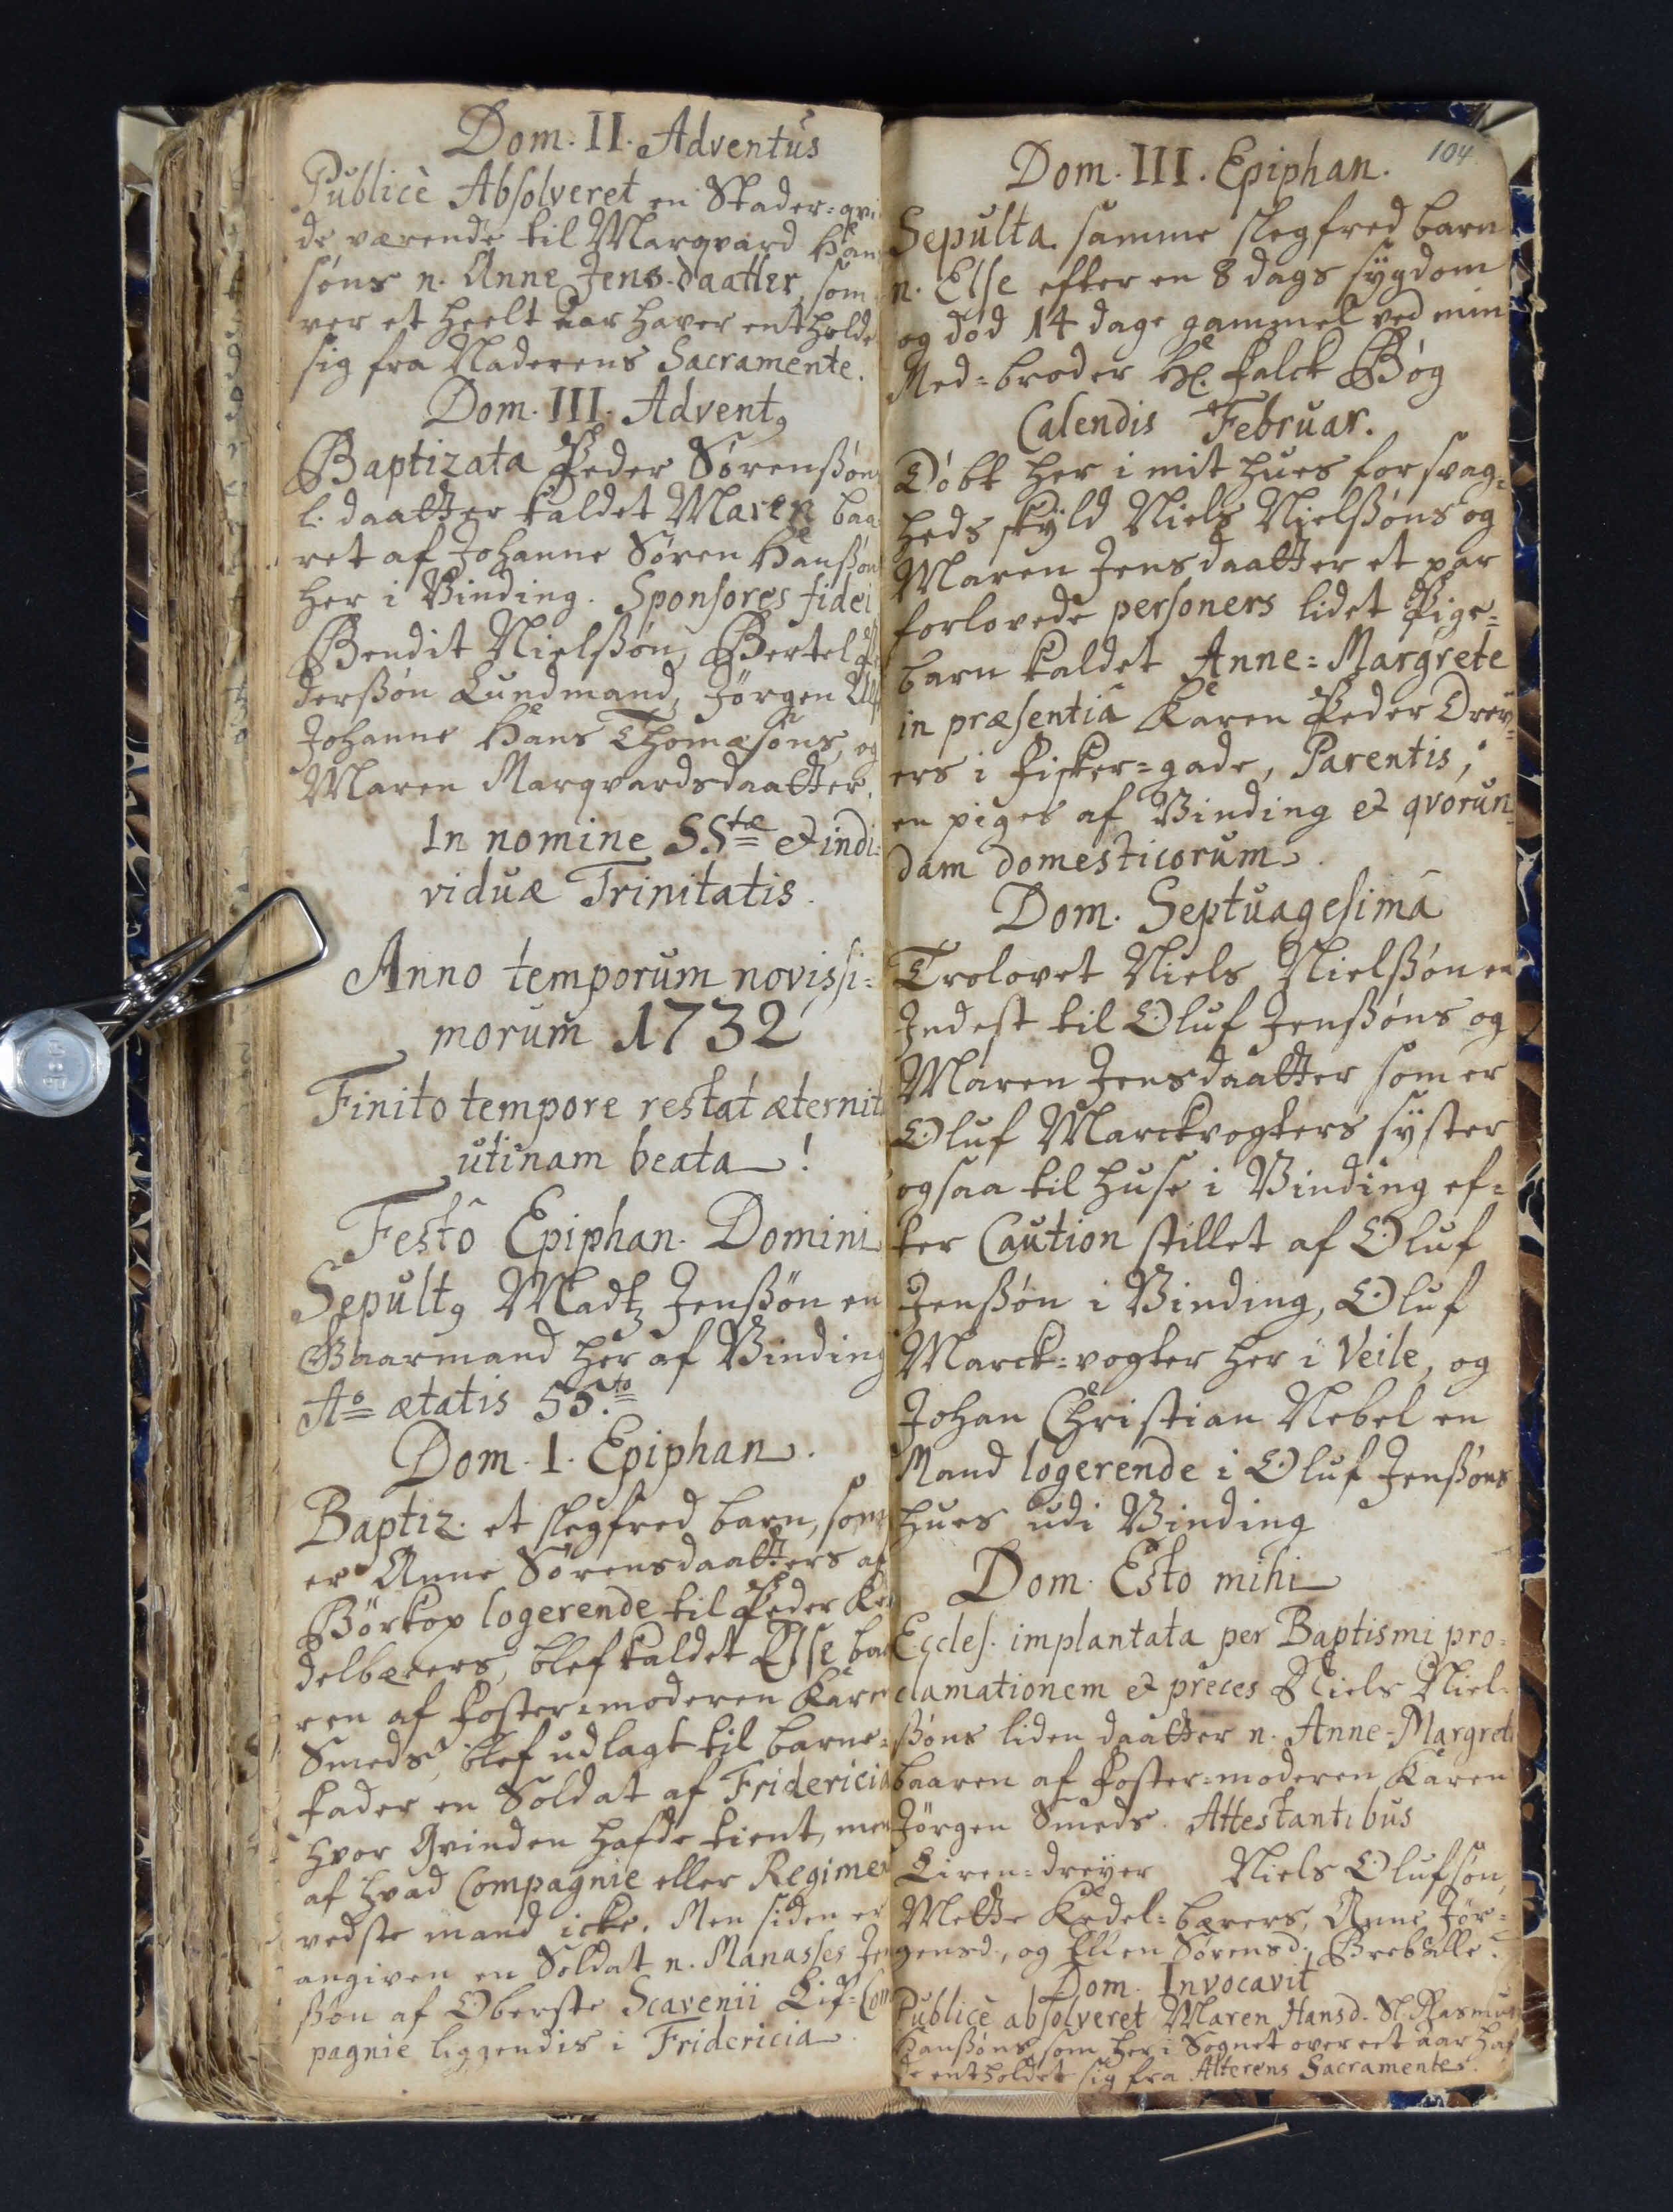Dom. II. Adventus
Publice Absolveret en Stader=qvi  
de værende til Marqvard Han
søns n. Anne Jens-daatter, som
ver et heelt Aar haver entholde  
sig fra Nadverens Sacramente.
Dom. III. Adventq
Baptizata Peder Sørenssøns
l. daatter kaldet Maren, baa¬
ret af Johanne Søren Hanssøn  
her i Vinding. Sponsores fidei
Bendit Nielssøn. Bertel Pe
derssøn Lundmand, Jørgen Ulf  
Johanne Hans Thomæsøns, og
Maren Marqvardsdaatter.
In nomine SStæ et indi¬
viduæ Trinitatis.
Anno temporum novissi¬
morum 1732
Finito tempore restat æternit
utinam beata!
Festo Epiphan. Domini
Sepultq Madtz Jenssøn en
Gaardmand her af Vinding
Ao ætatis 55to.
Dom. I. Epiphan.
Baptiz. et slegfred barn, som
er Anne Sørensdaatters af
Børcop logerende til Peder Ke  
delbærers, blef kaldet Else, bar  
ren af Foster=moderen Kare  
Smeds, blef udlagt til barne
fader en Soldat af Fridericia  
hvor Qvinden hafde tient, men  
af hvad Compagnie eller Regime  
vedste mand icke. Men siden er
angiven en Soldat n. Manasses Je  
ssøn af Oberste Scavenii Lif=Com  
pagnie liggendis i Fridericia
104
Dom. III. Epiphan.
Sepulta samme slegfred barn
n. Else, efter en 8 dages sygdom
og død 14 dage gammel ved min 
Med=broder Hl Falck Bøg
Calendis Februar.
Døbt her i mit hues for svag¬
heds skyld Niels Nielssøns og
Maren Jensdaatter et par
forlovede personers lidet Pige¬
barn kaldet Anne = Margrete
in præsentia Karen Peder Drey¬
ers i Fisker=gade, Parentis
en piges af Vinding et qvorun¬
dam domesticorum.
Dom. Septuagesima
Trolovet Niels Nielssøn en
Indest til Oluf Jenssøns og
Maren Jensdaatter som er
Oluf Marckvogters syster
ogsaa til huse i Vinding ef¬
ter Caution stillet af Oluf
Jenssøn i Vinding, Oluf
Marck=vogter her i Veile, og
Johan Christian Nebel en
Mads logerende i Oluf Jenssøns
hues udi Vinding
Dom. Esto mihi
Eccles. implantata per Baptismi pro¬
clamationem et preces Niels Niel¬
ssøns liden daatter n. Anne-Margret  
baarent af Foster=moderen Karen
Jørgen Smeds. Attestantibus
Liren=dreyer Niels Olufsøn,
Mette Kedel=bærers, Anne Jør¬
gensd., og Ellen Sørensd. Breballe.
Dom. Invocavit
Publice absolveret Maren Hansd. Sl. Rasmus
Hanssøns som her i Sognet over eet Aar haf
de entholdet sig fra Alterens Sacramente.
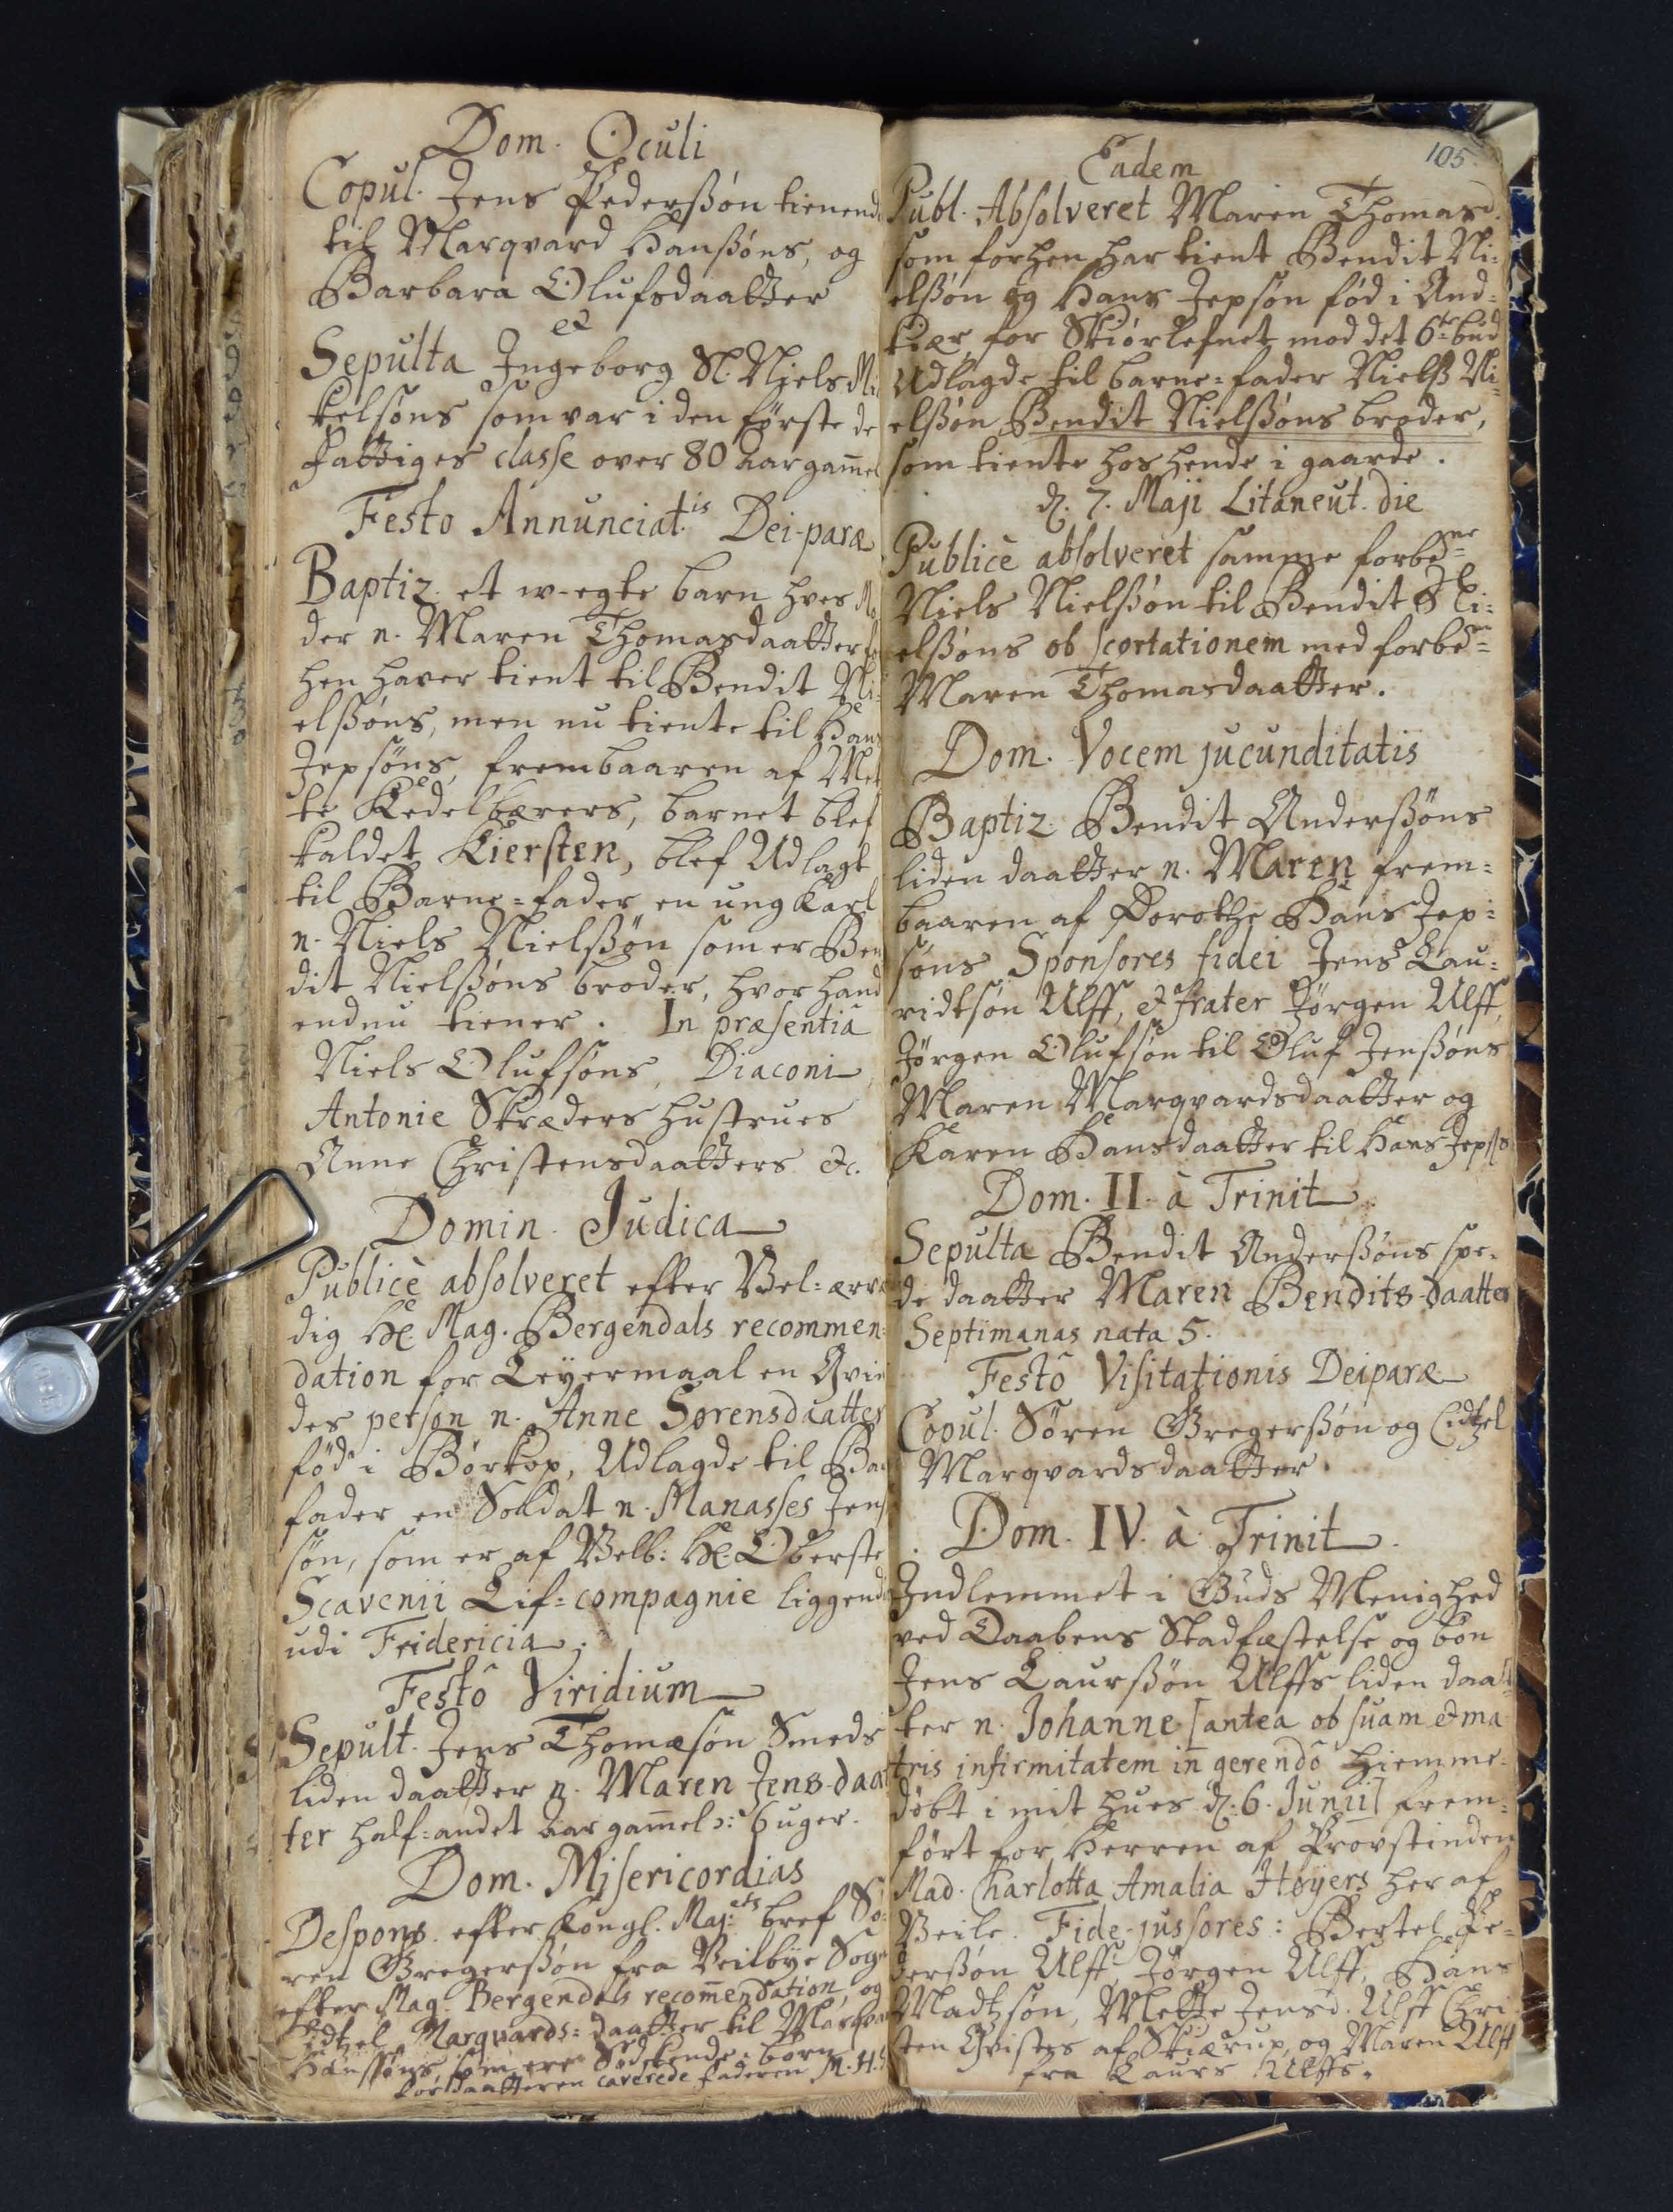Dom. Oculi
Copul. Jens Pederssøn tienende  
til Marqvard Hanssøns, og
Barbara Olufsdaatter.
et
Sepulta Ingeborg Sl. Niels Mi  
kelsøns som var i den første de 
fattiges classe over 80 Aar gammel
Festo Annunciatis Dei-paræ.
Baptiz. et w-egte barn hves Mo
der n. Maren Thomasdaatter fo  
hen haver tient til Bendit Ni¬
elssøns, men nu tiente til Hans
Jepsøns, frembaaren af Met
te Kedelbærers, barnet blef
kaldet Kiersten, blef Udlagt
til Barne=fader en ung Karl
n. Niels Nielssøn som er Ben
dit Nielssøns broder, hvor hand
endnu tiener. In præsentia
Niels Olufsøns, Diaconi
Antonie Skræders hustrues
Anne Christensdaatters etc.
Domin Judica
Publice absolveret efter Vel=ærve  
dig Hl. Mag. Bergendals recommen¬
dation for Leyermaal en Qvin
des person n. Anne Sørensdaatter
fød i Børcop. Udlagde til Bar  
fader en Soldat n. Manasses Jens
søn, som er af Velb: Hl Oberste
Scavenii Lif=Compagnie liggendis  
udi Fridericia.
Festo Viridium
Sepult. Jens Thomæsøn Smeds
liden daatter n. Maren Jens-daat¬  
ter half=andet Aar gamel ↄ: 6 uger.
Dom. Misericordias
Despons. efter Kongl. Majets bref Sø¬
ren Gregerssøn fra Veilbye Sogn  
efter Mag. Bergerdals recomendation, og
Cidtzel Marqvards=daatter til
Hanssøns, som ere Sødskende = børn
for daatteren caverede Faderen M.H.S
105.
Eadem
Publ. Absolveret Maren Thomasd.
som forhen har tient Bendit Ni¬
elssøn og Hans Jepsøn fød i And¬
kiær, for Skiørlefnet mod det 6te bud
Udlagde til barne=fader Niels Ni¬
elssøn Bendit Nielssøns broder,
som tiente hos hende i gaarde.
d. 7. Maji Litaneut. die
Publice absolveret samme forbene
Niels Nielssøn til Bendit Ni¬
elsøns ob scortationem med forbe##
Maren Thomasdaatter.
Dom. Vocem jucunditatis
Baptiz. Bendit Andersøns
liden daatter n. Maren frem¬
baaren af Dorothe Hans Jep¬
søns Sponsores fidei Jens Lau¬
ridtsøn Ulff, et frater Jørgen Ulff,
Jørgen Olufsøn til Oluf Jenssøns
Maren Marqvardsdaatter og
Karen Hansdaatter til Hans Jepss
Dom. II. a Trinit
Sepulta Bendit Anderssøns spæ¬
de daatter Maren Bendits-daatter
Septimanas nata 5.
Festo Visitationis Deiparæ
Copul. Søren Gregerssøn og Cidtzel
Marqvardsdaatter.
Dom. IV. a Trinit
Indlemmet i Guds Menighed
ved Daabens Stadfæstelse og bøn
Jens Laurssøn Ulffs liden daat¬
ter n. Johanne [antea ob suam et ma¬
tris infirmitatem in gerendo Hiemme¬
døbt i mit hues d. 6. Junii] frem¬
ført for Herren af Provstinden
Mad. Charlotta Amalia Høyers her af
Veile. Fide-jussortes: Bertel Pe¬
dersøn Ulff, Jørgen Ulff, Hans
Madtzsøn, Mette Jensd. Ulff Chri
sten Qvistes af Skiærup, og Maren Ulff
fra Laurs Ulffs.